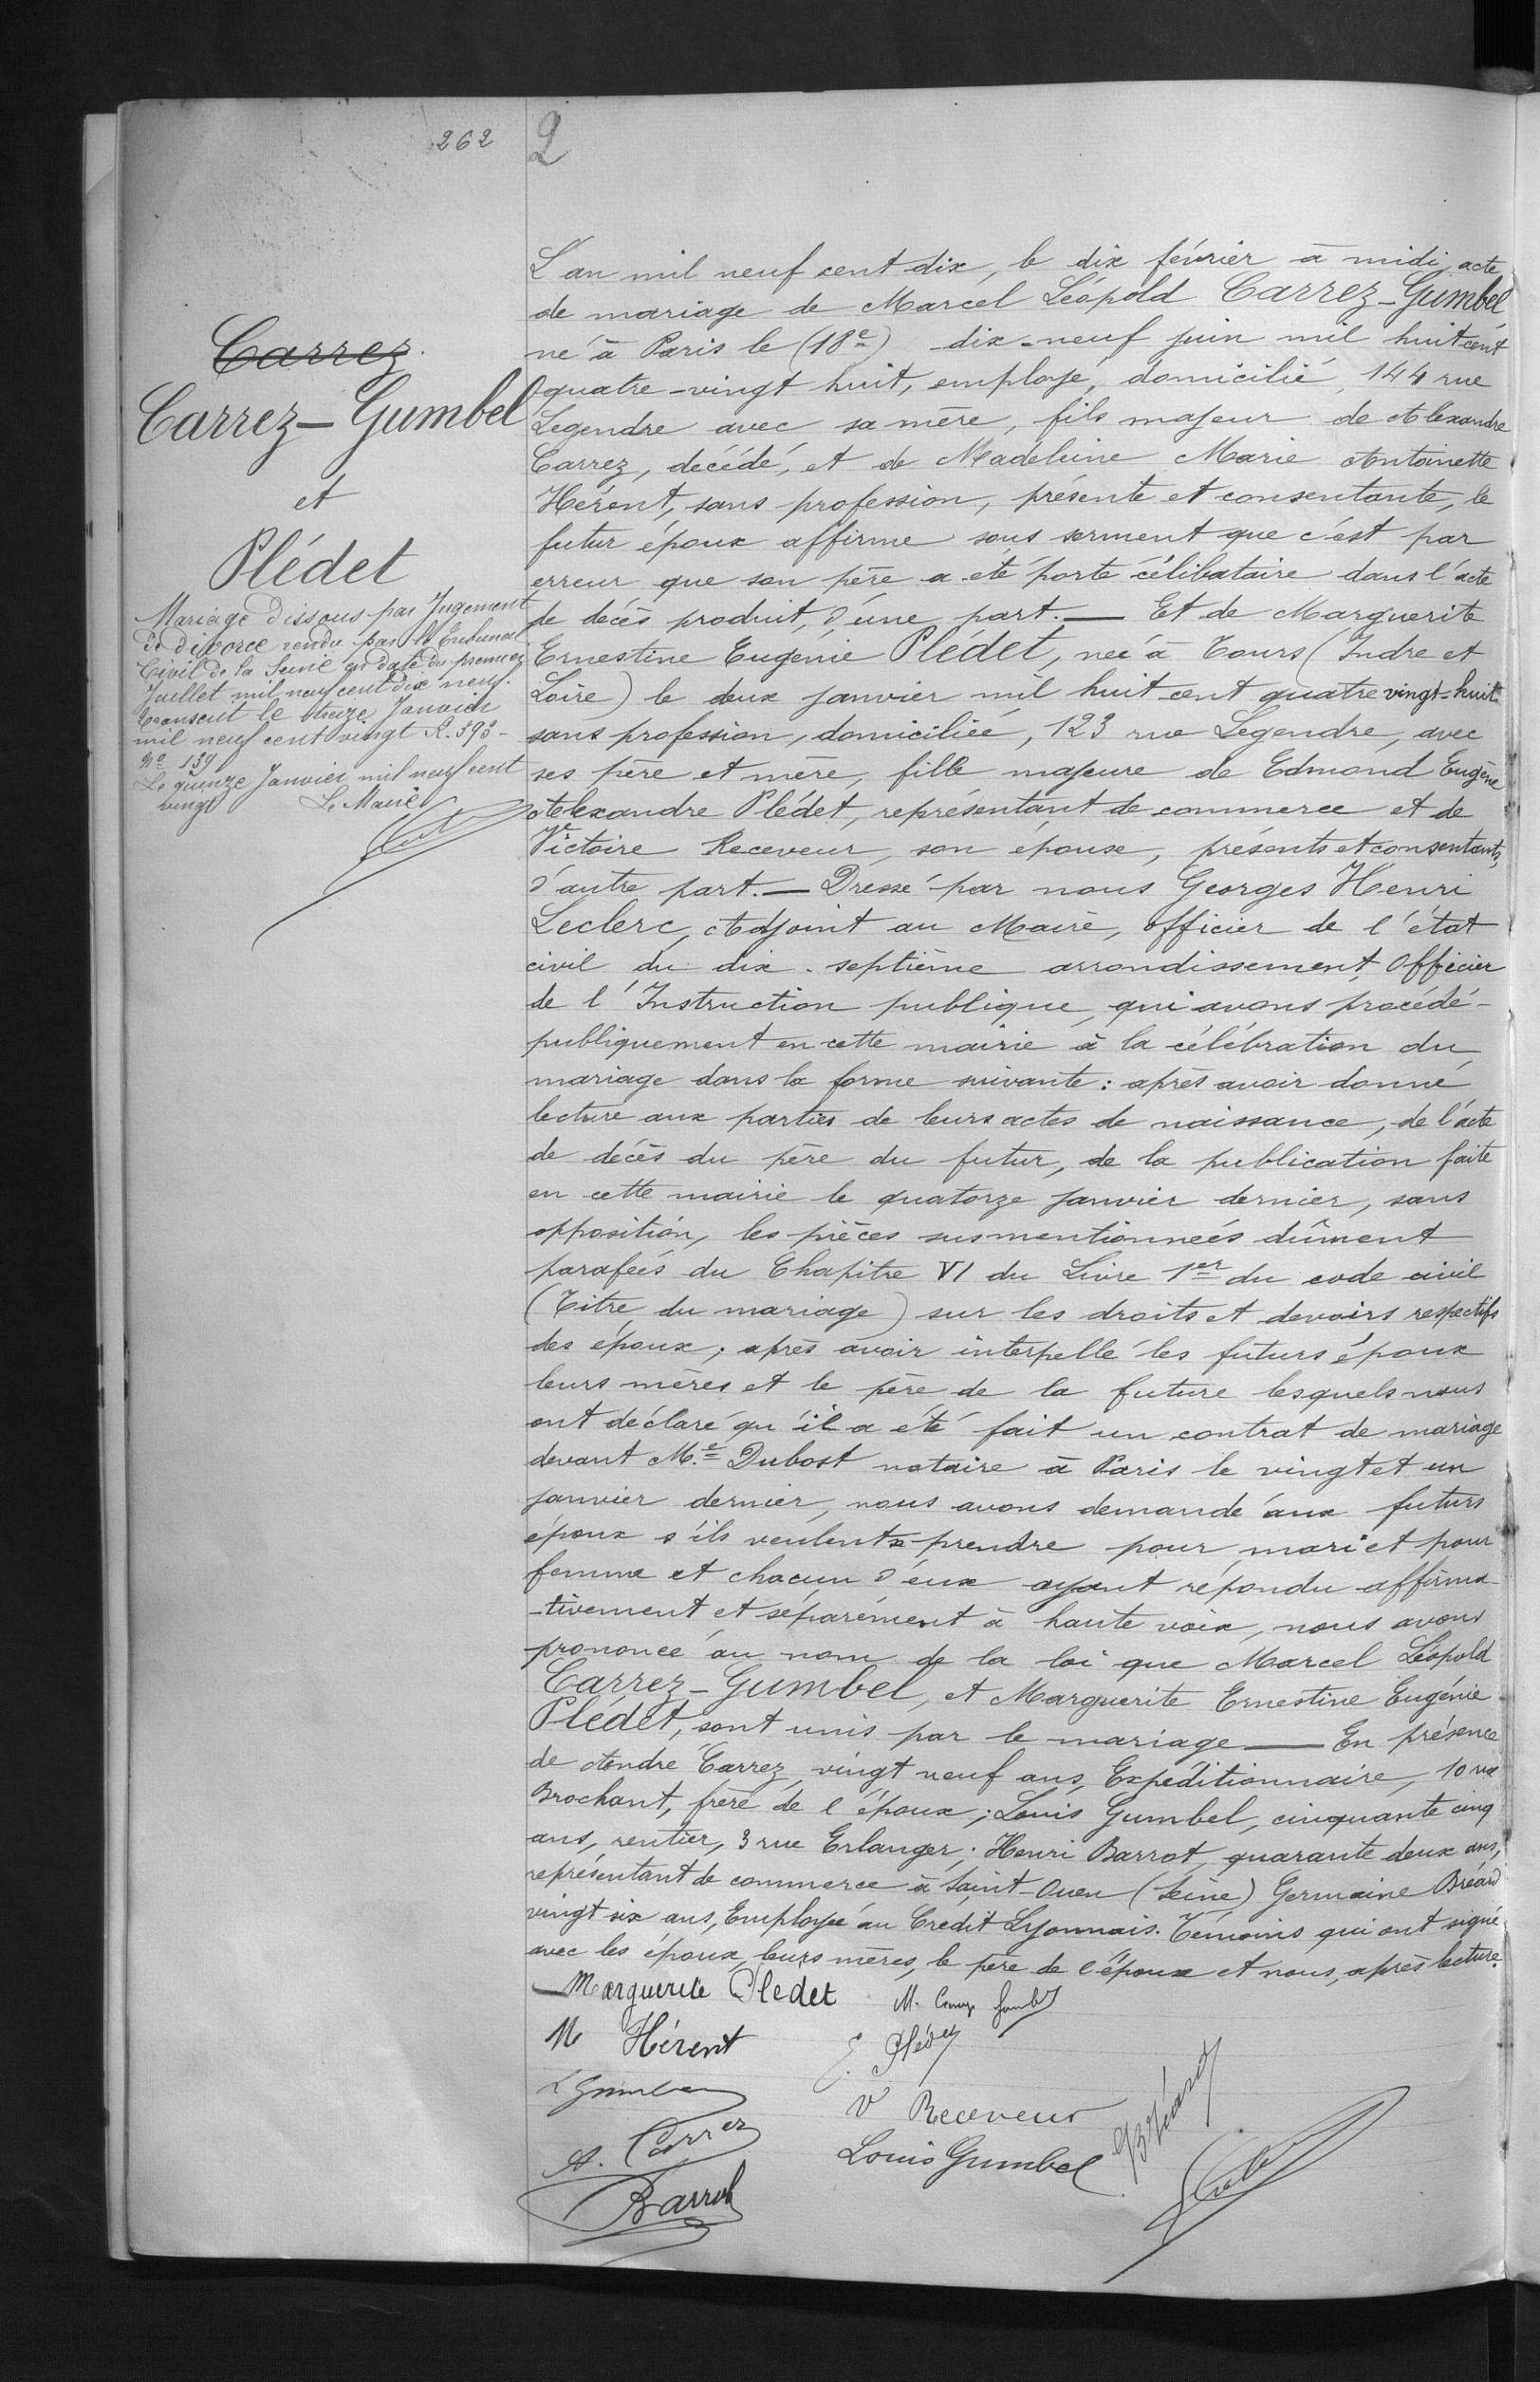262
Carrez-Gumbel
et
Plédet
L'an mil neuf cent dix, le dix février à midi acte
de mariage de Marcel Léopold Carrez-Gumbel
né à Paris le (18e) dix-neuf juin mil huit cent
quatre-vingt huit, employé, domicilié, 144 rue
Legendre avec sa mère, fils majeur de Alexandre
Carrez, décédé, et de Madeleine Marie Antoinette
Héront, sans profession, présente et consentante, le
futur époux affirme sous serment que c'est par
erreur que son père a été porté célibataire dans l'acte
de décès produit d'une part. -Et de Marguerite
Ernestine Eugénie Plédet, née à Tours (Indre et
Loire) le deux janvier mil huit cent quatre vingt-huit
sans profession, domicilié, 123 rue Legendre, avec
ses père et mère, fille majeure de Edmond Eugène
Alexandre Plédet, représentant de commerce et de
Victoire Receveur, son épouse, présents et consentants,
d'autre part.-Dressé par nous Georges Henri
Leclerc, Adjoint au Maire, officier de l'état
civil du dix-septième arrondissement Officier
de l'Instruction publique, qui avons procédé-
publiquement en cette mairie à la célébration du
mariage dans la forme suivante: après avoir donné
lecture aux parties de leurs actes de naissance, de l'acte
de décès du père du futur, de la publication faite
en cette mairie le quatorze janvier dernier, sans
opposition, les pièces susmentionnées dûment
parafés du Chapitre VI du Livre 1er du code civil
(Titre du mariage) sur les droits et devoir respectifs
des époux, après avoir interpellé les futurs époux
leur mères et le père de la futur lesquels nous
ont déclaré qu'il a été fait un contrat de mariage
devant M° Dubort notaire à Paris le vingt et un
janvier dernier, nous avons demandé aux futurs
époux s'ils veulent se prendre pour mari et pour
femme et chacun d'eux ayant répondu affirma-
tivement et séparément à haute voix, nous avons
prononcé au nom de la loi que Marcel Léopold
Carrez-Gumbel, et Marguerite Ernestine Eugénie
Plédet, sont unis par le mariage -En présence
de André Carrez, vingt neuf ans, Expéditionnaire, 10 rue
Brochant, frère de l'époux; Louis Gumbel, cinquante cinq
ans, rentier, 3 rue Erlanger Henri Barrot, quarante deux ans,
représentant de commerce à Saint-Ouen (Seine) Germaine Bréard
vingt six ans, Employée au Crédit Lyonnais. Témoins qui ont signé
avec les époux, leurs mères, le père de l'époux et nous, après lecture.
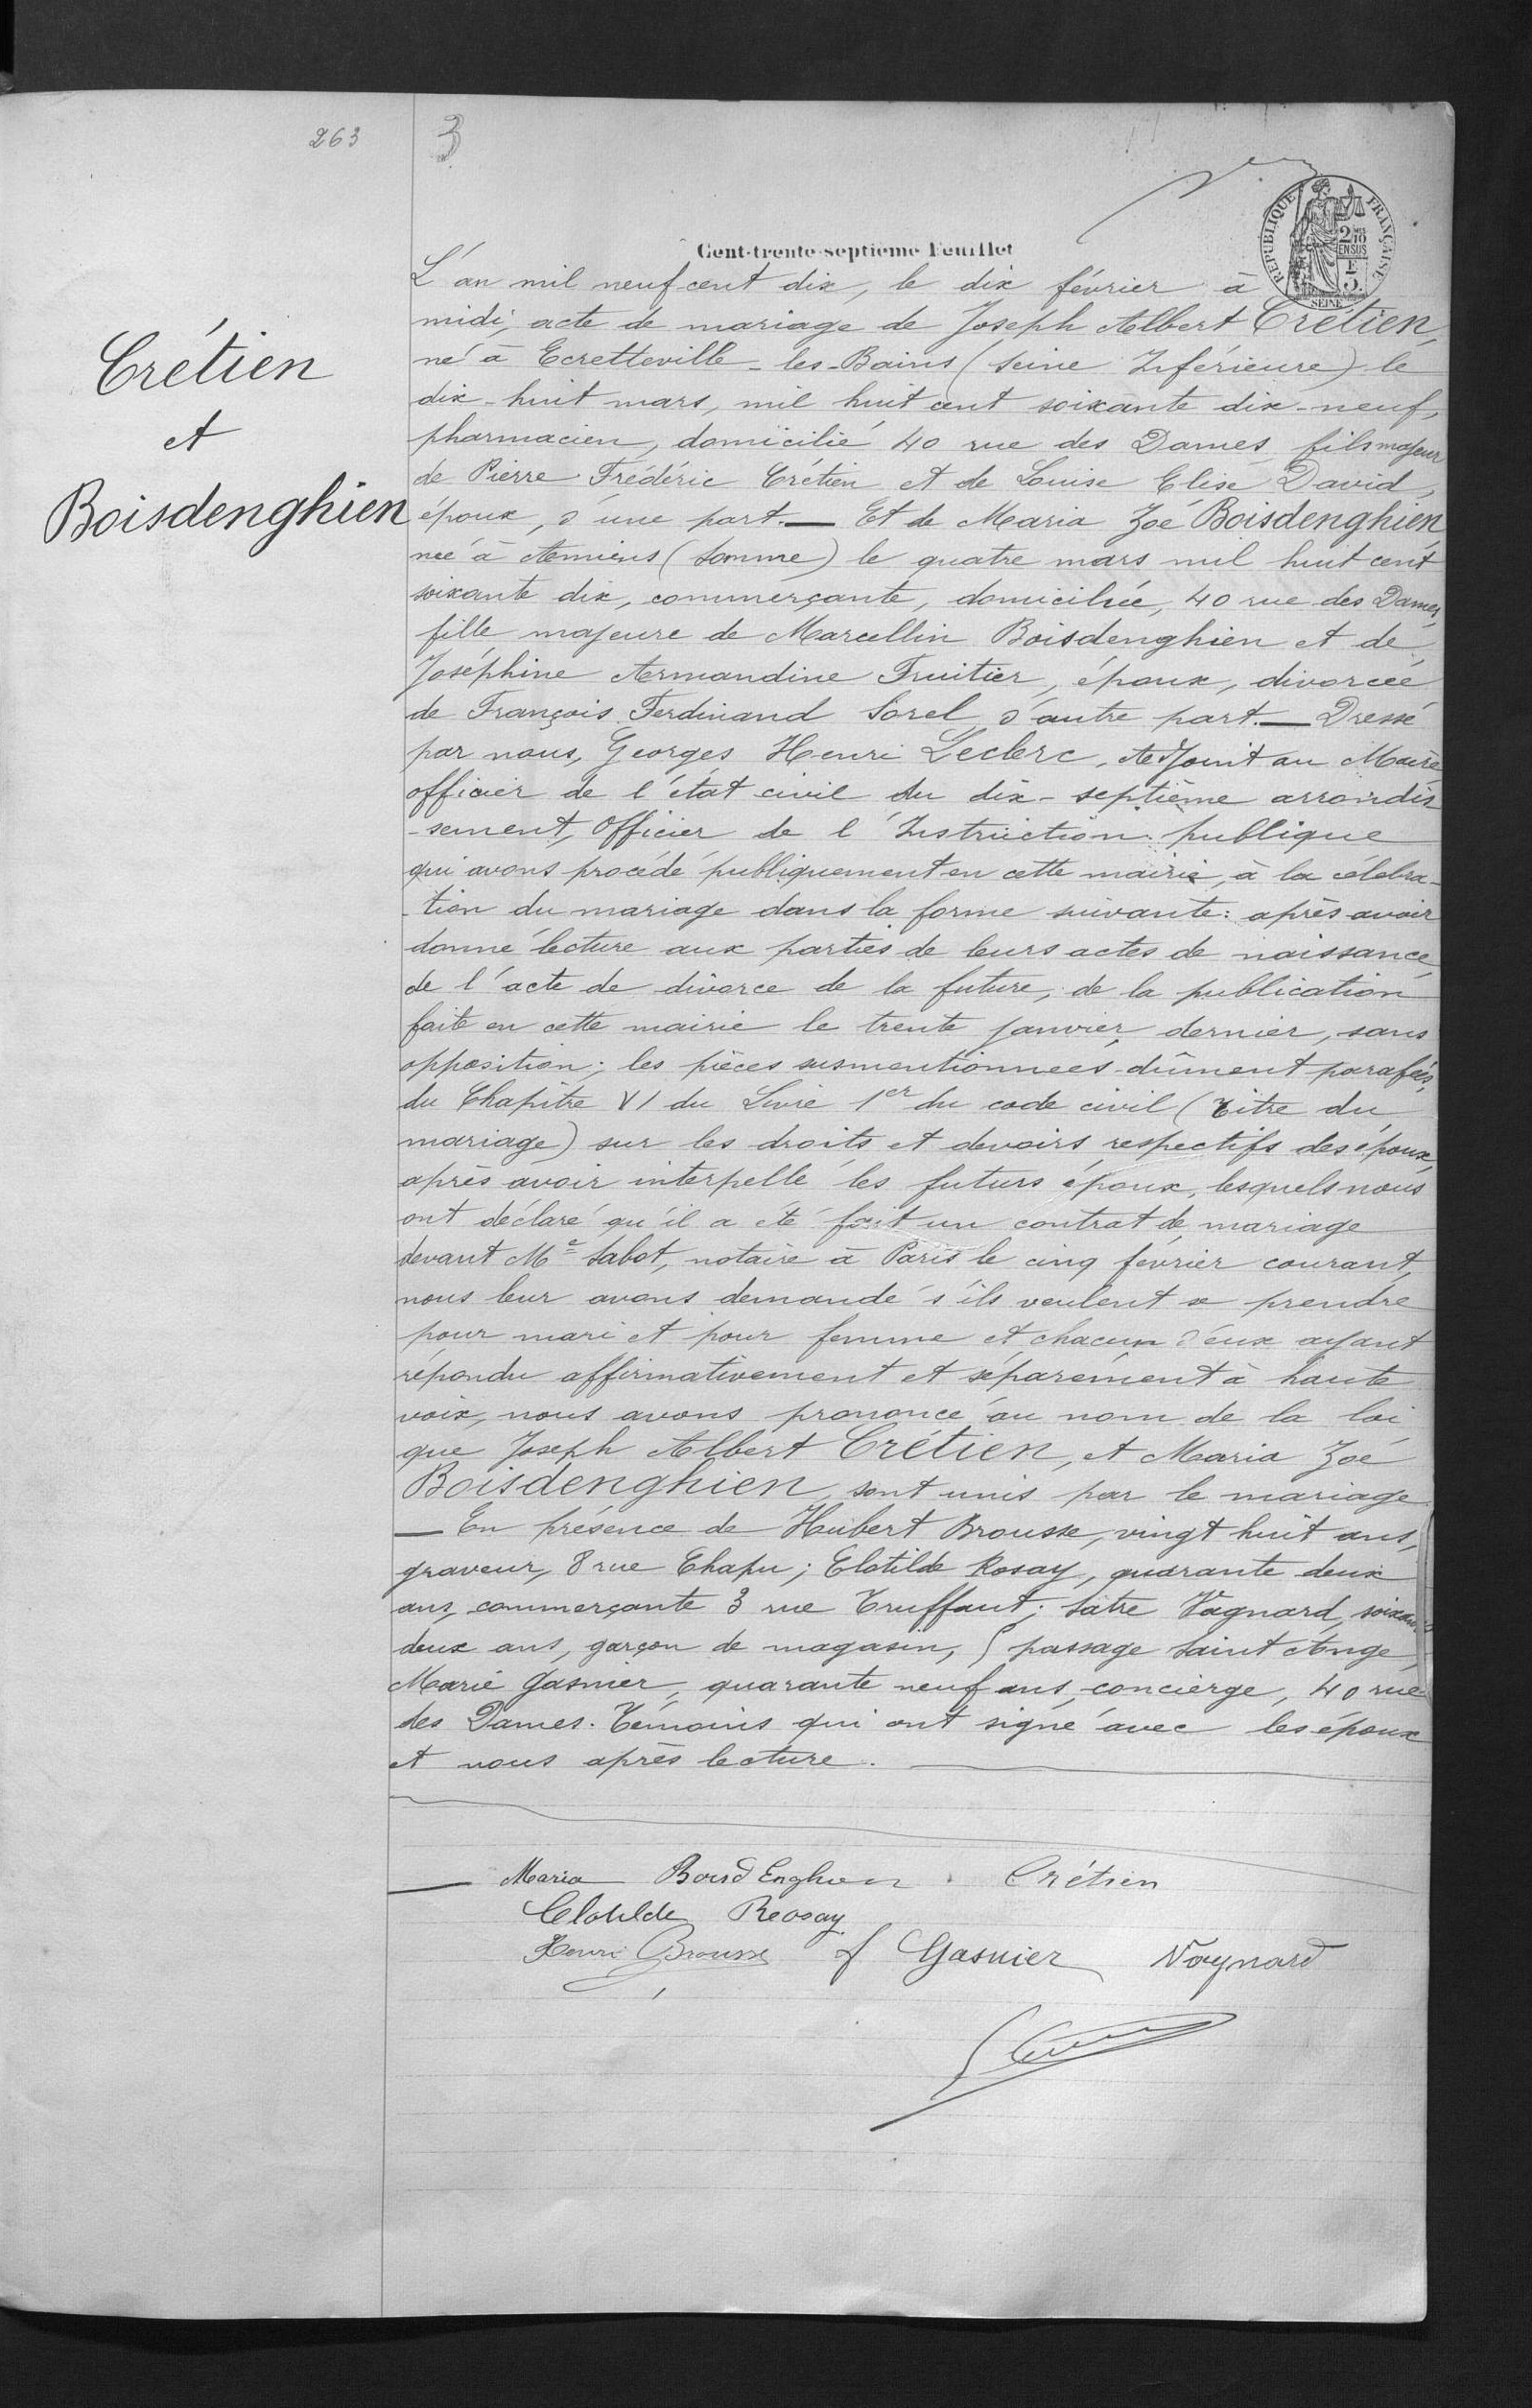263
Crétien
et
Boisdenghien
L'an mil neuf cent dix, le dix février à
midi, acte de mariage de Joseph Albert Crétien
né à Ecretteville-les-Bains (Seine Inférieure) le
dix-huit mars, mil huit cent soixante dix-neuf,
pharmacien, domicilié 40 rue des Dames, fils majeur
de Pierre Frédéric Crétien et de Louise Elise David,
époux, d'une part-Et de Maria Zoé Boisdenghien
née à Arniers (Seomme) le quatre mars mil huit cent
soixante dix, commerçante, domiciliée, 40 rue des Dames,
fille majeure de Marcellin Boisdenghien et de
Joséphine Armandine Fruitier, époux, divorcée
de François Ferdinand Sorel d'autre part -Dressé
par nous, Georges Henri Leclerc. Adjoint au Mairie
officier de l'état civil du dix-septième arrondi
-sement officier de l'Instruction publique
qui avons procédé publiquement en cette mairie à la célébra-
tion du mariage dans la forme suivante: après avoir
donné lecture aux parties de leurs actes de naissance
de l'acte de divorce de la future, de la publication
fait en cette mairie le trente janvier dernier, sans
opposition: les pièces susmentionnées dûment parafées
du Chapitre VI du Livre 1er du code divil (Titre du
mariage) sur les droits et devoir respectifs des époux
après avoir interpellé les futurs époux lesquels nous
ont déclaré qu'il a été fait un contrat de mariage
devant M°Sabot, notaire à Paris le cinq février courant
nous leur avons demandé s'ils veulent se prendre
pour mari et pour femme et chacun d'eux ayant
répondu affirmativement et séparèment à haute
voix, nous avons prononcé au nom de la loi
que Joseph Albert Crétien, et Maria Zoé
Boisdenghien, sont unis par le mariage
-En présence de Hubert Brousse, vingt huit ans,
graveur, 8 rue Chapin; Clotilde Rosay, quarante deux
ans, commerçante 3 rue Truffaut; Satre Vagnard, soixan
deux ans, garçon de magasin, 5 passage Saint Ange
Marie Gasnier, quarante neuf ans, concierge, 40 rue
des Dames. Témoins qui ont signé avec les époux
et nous après lecture.-